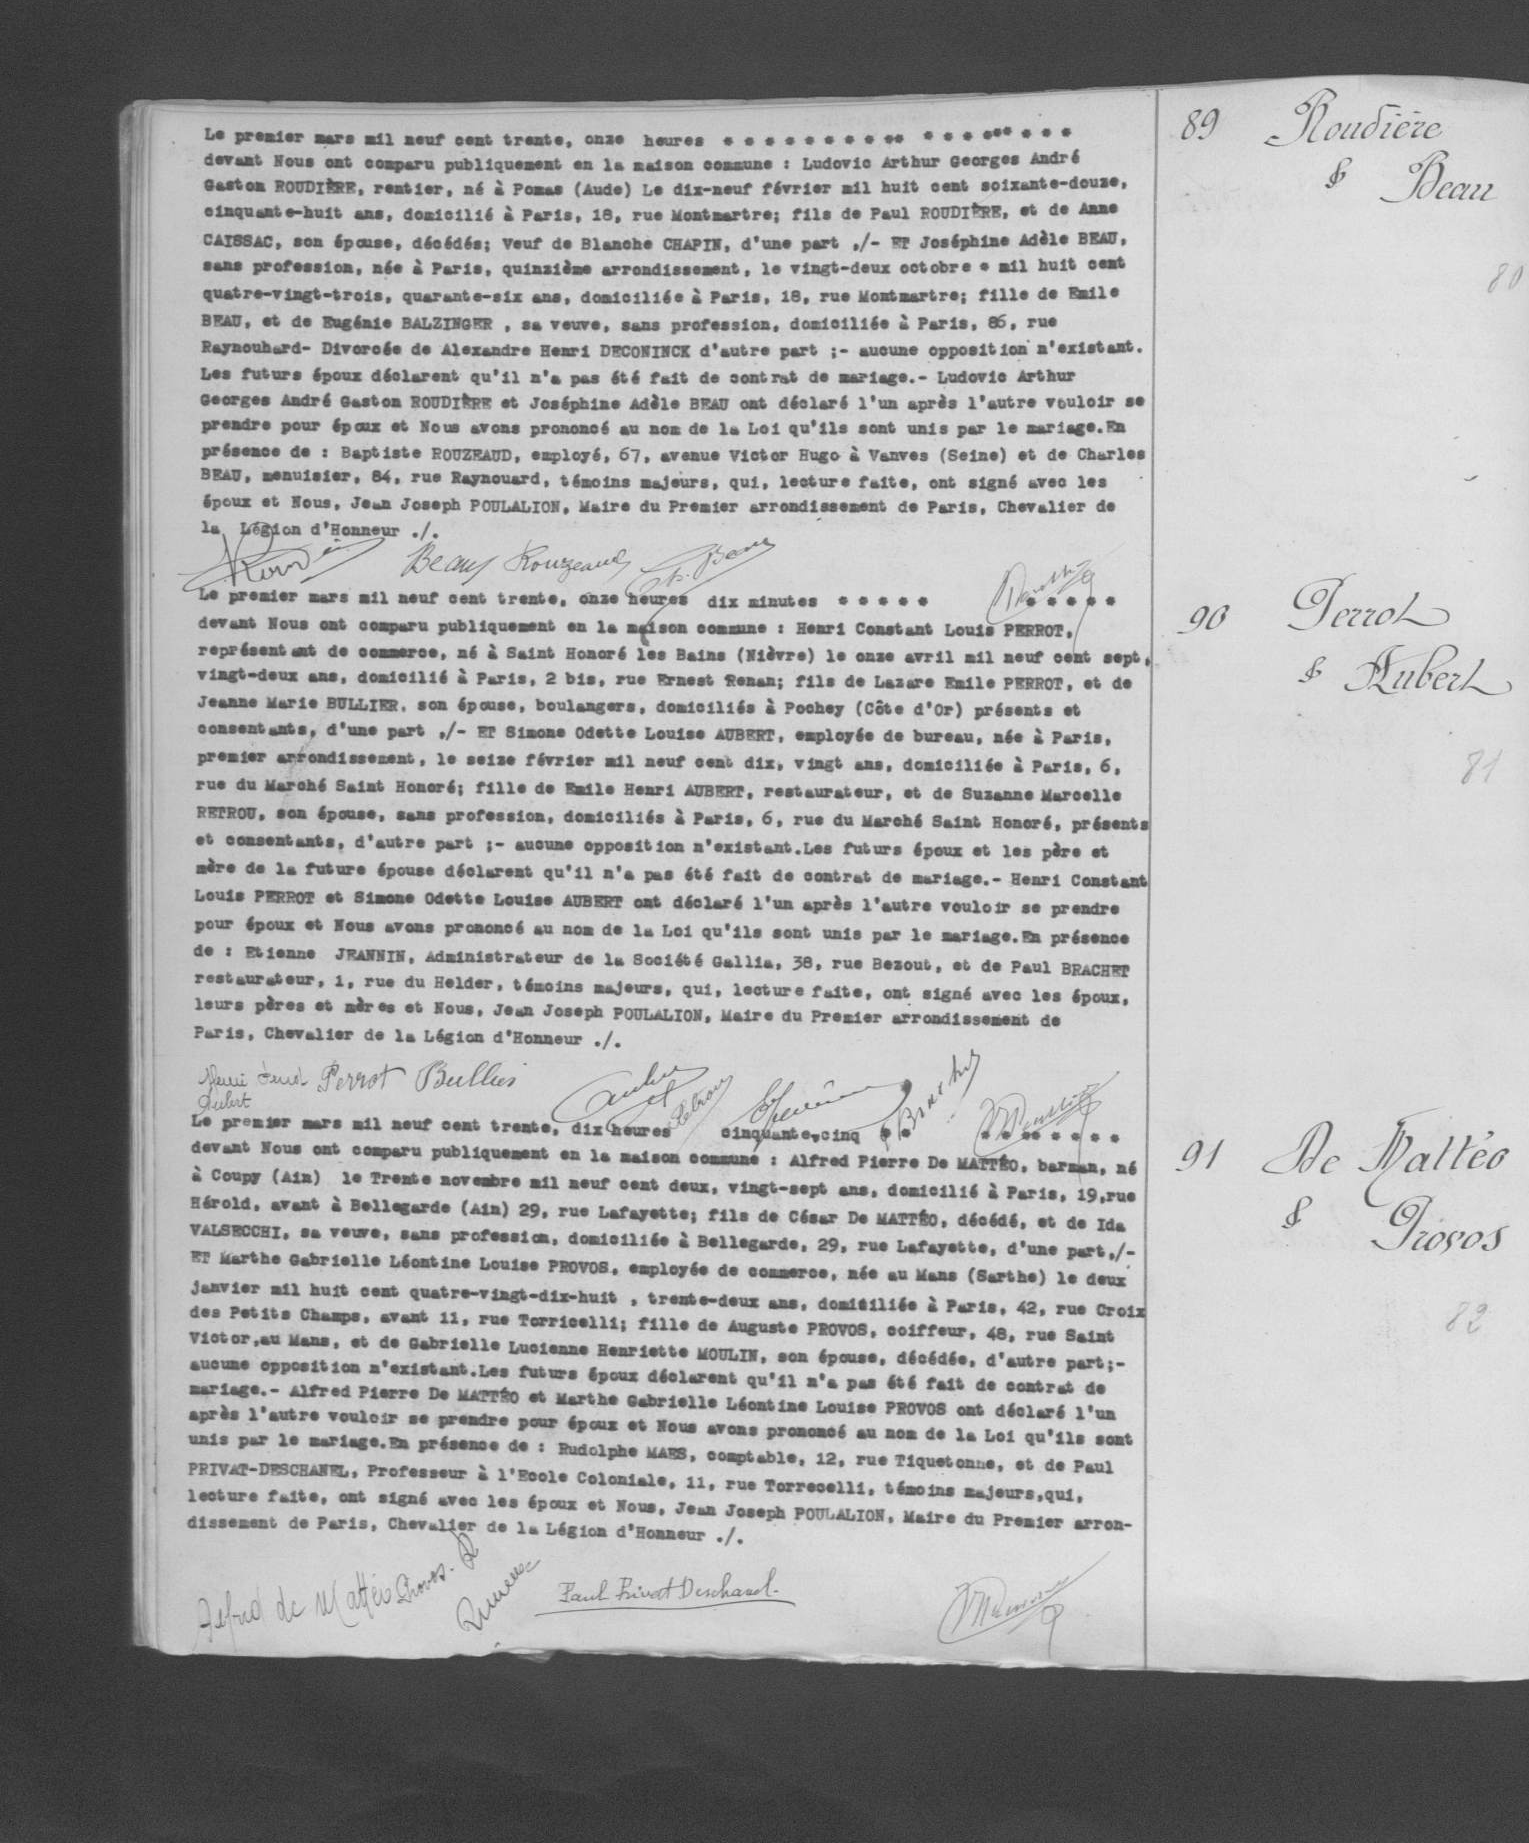Le premier mars mil neuf cent trente, onze heures ****
devant Nous ont comparu publiquement en la maison commune: Ludovic Arthur Georges André
Gaston ROUDIERE, rentier, né à Pomas (Aude) le dix-neuf février mil huit cent soixante-douze,
cinquante-huit ans, domicilié à Paris, 18, rue Montmarttre; fils de Paul ROUDIERE, et de Anne
CAISSAC, son épouse, décédés; Veuf de Blanche CHAPIN, d'une part ,/-ET Joséphine Adèle BEAU,
sans profession, née à Paris, quinzième arrondissement, le vingt-deux octobre * mil huit cent
quatre-vingt-trois, quarante-six ans, domiciliée à Paris, 18, rue Montmartre; fille de Emile
BEAU, et de Eugénie BALZINGER, sa veuve, sans profession, domiciliée à Paris, 86, rue
Raynouhard-Divorcée e Alexandre Henri DECONINCK d'autre part ;-aucune opposition n'existant.
Les futurs époux déclarent qu'il n'a pas été fait de contrat de mariage .-Ludovic Arthur
Georges André Gaston ROUDIERE et Joséphine Adèle BEAU ont déclaré l'un après l'autre vouloir se
prendre pour époux et Nous avons prononcé au nom de la Loi qu'ils sont unis par le mariage. En
présence de: Baptiste ROUZEAUD, employé, 67, avenue Victor Hugo à Vanves (Seine) et de Charles
BEAU, menuisier, 84, rue Raynouard, témoins majeurs, qui, lecture faite, ont signé avec les
époux et Nous, Jean Joseph POULALION, Maire du Premier arrondissement de Paris, Chevalier de
la Légion d'Honneur ./.
Le premier mars mil neuf cent trente, onze heures dix minutes ****
devant Nous ont comparu publiquement en la maison commune: Henri Constant Louis PERROT,
représentant de commerce, né à Saint Honoré les Bains (Nièvre) le onze avril mil neuf cent sept,
vingt-deux ans, domicilié à Paris, 2 bis, rue Ernest Renan; fils de Lazare Emile PERROT, et de
Jeanne Marie BULLIER, son épouse, boulangers, domiciliés à Pochey (Côte d'Or) présents et
consentants, d'une part ,/-ET Simone Odette Louise AUBERT, employée de bureau, née à Paris,
premier arrondissement, le seize février mil neuf cent dix, vingt ans, domiciliée à Paris, 6,
rue du Marché Saint Honoré; fille de Emile Henri AUBERT, restaurateur, et de Suzanne Marcelle
RETROU, son épouse, sans profession, domiciliés à Paris, 6, rue du Marché Saint Honoré, présents
et consentants, d'autre part ;-aucune opposition n'existant. Les futurs époux et les père et
mère de la future épouse déclarent qu'il n'a pas été fait de contrat de mariage .-Henri Constant
Louis PERROT et Simone Odette Louise AUBERT ont déclaré l'un après l'uatre vouloir se prendre
pour époux et Nous avons prononcé au nom de la Loi qu'ils sont unis par le mariage. En présence
de: Etienne JEANNIN, Administrateur de la Société Gallia, 38, rue Bezout, et de Paul BRACHET
restaurateur, 1, rue du Helder, témoins majeurs, qui, lecture faite, ont signé avec les époux,
leurs pères et mères et Nous, Jean Joseph POULALION, Maire du Premier arrondissement de
Paris, Chevalier de la Légion d'Honneur ./.
Le premier mars mil neuf cent trente, dix heures cinquante-cinq ****
devant Nous ont comparu publiquement en la maison commune: Alfred Pierre De MATTEO, barman, né
à Coupy (Ain) le Trente novembre mil neuf cent deux, vingt-sept ans, domicilié à Paris, 19, rue
Hérold, avant à Bellegarde (Ain), 29, rue Lafayette; fils de César De MATTEO, décédé, et de Ida
VALSECCHI, sa veuve, sans profession, domiciliée à Bellegarde, 29, rue Lafayette, d'une part ,/-
ET Marthe Gabrielle Léontine Louise PROVOS, employée de commerce, née au Mans (Sarthe) le deux
janvier mil huit cent quatre-vingt-dix-huit, trente-deux ans, domiciliée à Paris, 42, rue Croix
des Petits Champs, avant 11, rue Torricelli; fille de Auguste PROVOS, coiffeur, 48, rue Saint
Victor, au Mans, et de Gabrielle Lucienne Henriette MOULIN, son épouse, décédée, d'autre part ;-
aucune opposition n'existant. Les futurs époux déclarent qu'il n'a pas été fait de contrat de
mariage .-Alfred Pierre De MATTEO et Marthe Gabrielle Léontine Louise PROVOS ont déclaré l'un
après l'autre vouloir se prendre pour époux et Nous avons prononcé au nom de la Loi qu'ils sont
unis par le mariage. En présence de: Rudolphe MAES, comptable, 12, rue Tiquetonne, et de Paul
PRIVAT-DESCHANEL, Professeur à l'Ecole Coloniale, 11, rue Torrecelli, témoins majeurs, qui,
lecture faite, ont signé avec les époux et Nous, Jean Joseph POULALION, Maire du Premier arron-
dissement de Paris, Chevalier de la Légion d'Honneur ./.
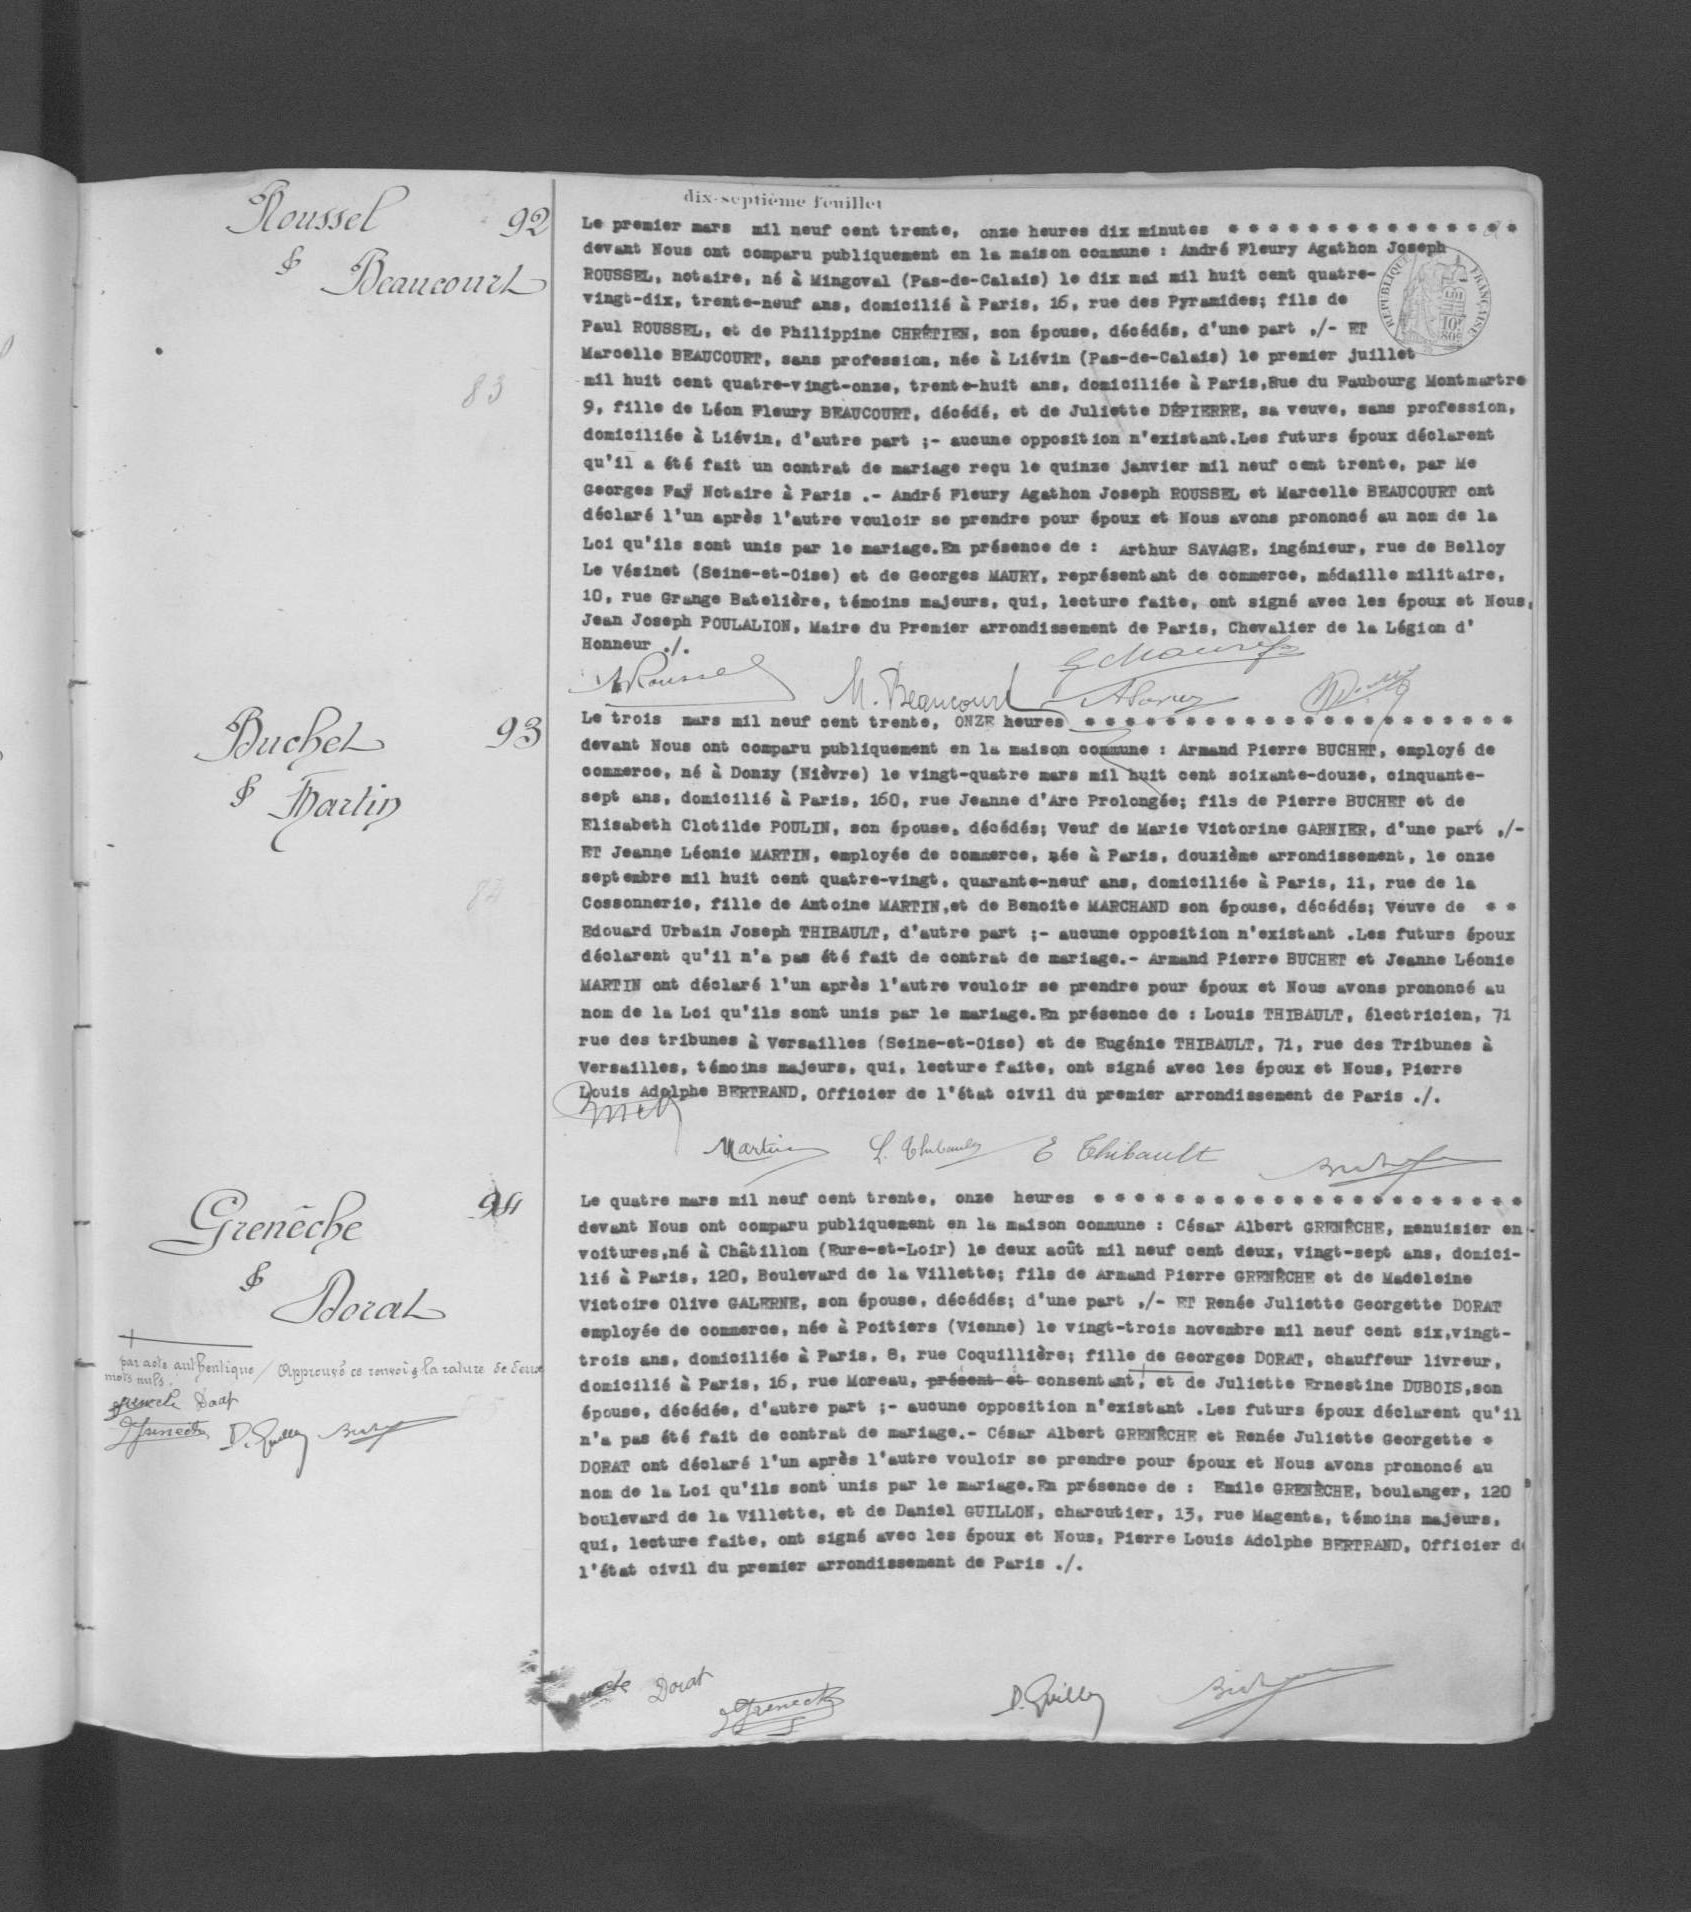Le premier mars mil neuf cent trente, onze heures dix minutes ****
devant Nous ont comparu publiquement en la maison commune: André Fleury Agathon Joseph
ROUSSEL, notaire, né à Mingoval (Pas-de-Calais) le dix mai mil huit cent quatre-
vingt-dix, trente-neuf ans, domicilié à Paris, 16, rue ds Pyramides; fils de
Paul ROUSSEL, et de Philippine CHRETIEN, son épouse, décédés, d'une part ,/-ET
Marcelle BEAUCOURT, sans profession, née à Liévin (Pas-de-Calais) le premier juillet
mil huit cent quatre-vingt-onze, trente-huit ans, domicilié à Paris, Rue du Faubourg Montmartre
9, fille de Léon Fleury BEAUCOURT, décédé, et de Juliette DEPIERRE, sa veuve, sans profession,
domiciliée à Liévin, d'autre part ;-aucune opposition n'existant. Les futurs époux déclarent
qu'il a été fait un contrat de mariage reçu le quinze janvier mil neuf cent trente, par Me
Georges Fay Notaire à Paris .-André Fleury Agathon Joseph ROUSSEL et Marcelle BEAUCOURT ont
déclaré l'un après l'autre vouloir se prendre pour époux et Nous avons prononcé au nom de la
Loi qu'ils sont unis par le mariage. En présence de: Arthur SAVAGE, ingénieur, rue de Belloy
Le Vésinet (Seine-et-Oise) et de Georges MAURY, représentant de commerce, médaille militaire,
10, rue Grange Batelière, témoins majeurs, qui, lecture faite ont signé avec les époux et Nous,
Jean Joseph POULALION, Maire du Premier arrondissement de Paris, Chevalier de la Légion d'
Honneur ./.
Le trois mars mil neuf cent trente, ONZE heures ****
devant Nous ont comparu publiquement en la maison commune: Armand Pierre BUCHET, employé de
commerce, né à Donzy (Nièvre) le vingt-quatre mars mil huit cent soixante-douze, cinquante-
sept ans, domicilié à Paris, 160, rue Jeanne d'Arc Prolongée; fils de Pierre BUCHET et de
Elisabeth Clotilde POULIN, son épouse, décédés; Veuf de Marie Victorine GARNIER, d'une part ,/-
ET Jeanne Léonie MARTIN, employée de commerce, née à Paris, douzième arrondissement, le onze
septembre mil huit cent quatre-vingt, quarante-neuf ans, domiciliée à Paris, 11, rue de la
Cossonnerie, fille de Antoine MARTIN, et de Benoite MARCHAND son épouse, décédés; Veuve de * *
Edouard Urbain Joseph THIBAULT, d'autre part ;-aucune opposition n'existant. Les futurs époux
déclarent qu'il n'a pas été fait de contrat de mariage .-Armand Pierre BUCHET et Jeanne Léonie
MARTIN ont déclaré l'un après l'autre vouloir se prendre pour époux et Nous avons prononcé au
nom de la Loi qu'ils sont unis par le mariage. En présence de: Louis THIBAULT, électricien, 71
rue des tribunes à Versailles (Seine-et-Oise) et de Eugénie THIBAULT, 71 rue des Tribunes à
Versailles, témoins majeurs, qui, lecture faite, ont signé avec les époux et Nous, Pierre
Louis Adolphe BERTRAND, Officier de l'état civil du premier arrondissement de Paris ./.
Le quatre mars mil neuf cent trente, onze heures ****
devant Nous ont comparu publiquement en la maison commune: César Albert GRENECHE, menuisier en
voitures, né à Châtillon (Eure-et-Loir) le deux août mil neuf cent deux, vingt-sept ans, domici-
lié à Paris, 120, Boulevard de la Villette; fils de Armand Pierre GRENECHE et de Madeleine
Victoire Olive GALERNE, son épouse, décédés; d'une part ,/-ET Renée Juliette Georgette DORAT
employée de commerce, née à Poitiers (Vienne) le vingt-trois novembre mil neuf cent six, vingt-
trois ans, domiciliée à Paris, 8, rue Coquillière; fille de Georges DORAT, chauffeur livreur,
domicilié à Paris, 16, rue Moreau, présent et consentant, et de Juliette Ernestine DUBOIS, son
épouse, décédée, d'autre part ;-aucune opposition n'existant. Les futurs époux déclarent qu'il
n'a pas été fait de contrat de mariage .-César Albert GRENECHE et Renée Juliette Georgette *
DORAT ont déclaré l'un après l'autre vouloir se prendre pour époux et Nous avons prononcé au
nom de la Loi qu'ils sont unis par le mariage. En présence de: Emile GRENECHE, boulanger, 120
boulevard de la Villette, et de Daniel GUILLON, charcutier, 13, rue Magenta, témoins majeurs,
qui, lecture faite, ont signé avec les époux et Nous, Pierre Louis Adolphe BERTRAND, Officier de
l'état civil du premier arrondissement de Paris ./.